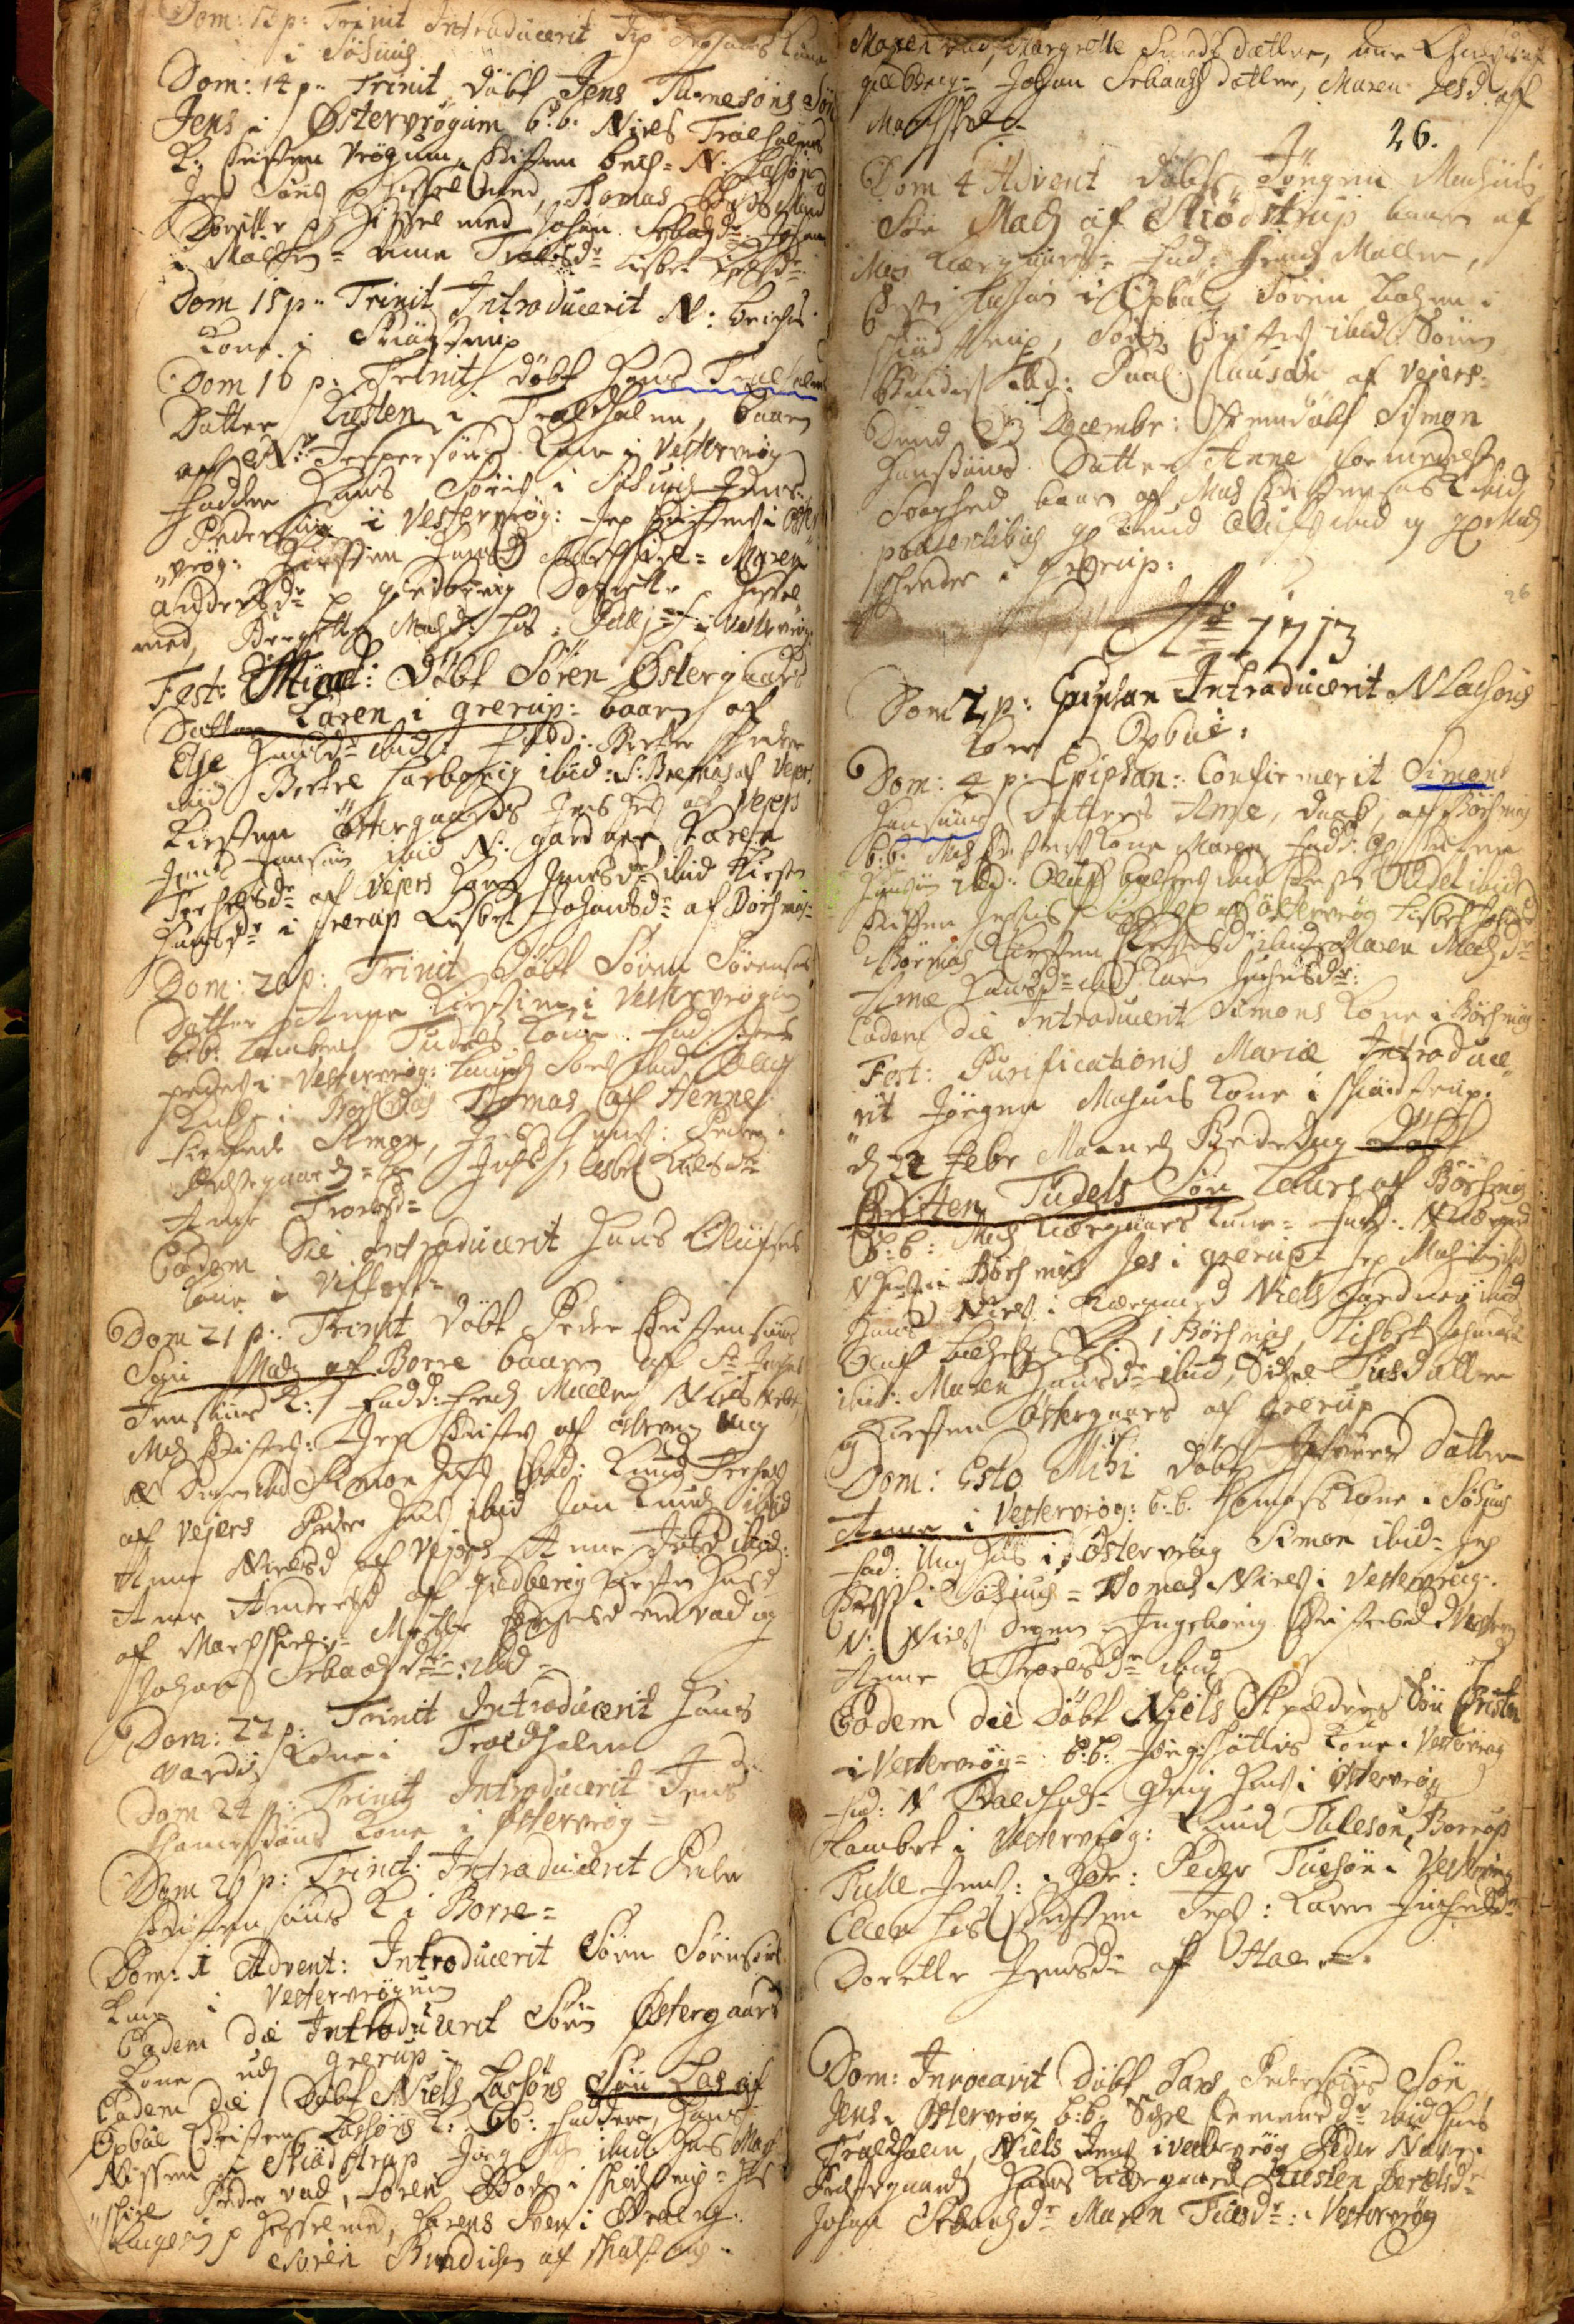Dom: 13 p: Trinit Introducerit Jep Jepsøns Kone
i Søhuus.
Dom: 14 p: Trinit døbt Jens Thomesøns Søn
Jens i Østervrøgum b.b. Niels Troldholms
K: Christen Vrøgum, Christen Bech: N: Lassøn
Jep Sørens p Hisselmed, Thomas Bodz Mand
Dorette p Hissel med, Johan Sebadzdr. Johanne
i Møllen: Anne Trolsdr Lisbet Kielsdr
Dom 15 p.. Trinit Introducerit N: Brichs
Kone i Skiødstrup
Dom 16 p: Trinit. døbt Hans Trolholms
Datter Kiesten i Troldholm, baaren
af N: Jespersøns Kone i Vestervrøg
Fadder Hans Sørens i Søhuus, Jens
Pedersøn i Vestervrøg: Jep Christensøn i Øster¬
vrøg: Kirsten Hansdr Marckschiel: Maren
Andersdr p Giedberig Dorette p Hissel¬
med, Bergitte Madzdr hos Pallj ## Vestervrøg:
Fest: Micael: Døbt Søren Østergaards
Datter Karen i Grerup: baaren af
Else Hansdr ibid: Fadd: Bertel Schreder
ibid Bertel Harborig ibid: S: Bremos af Vejers
Kirsten Østergaards Jes ## af Vejers
Jens Jensøn ibid N: Garders Karen
Terchelsdr af Vejers Karen Jensdr ibid Kirsten
Hansdr i Grerup, Lisbet Johansdr af Børsmøs:
Dom: 20 p: Trinit døbt Søren Sørensens
Datter Anne Kirstine i Vestervrøgum
b:b: Lambert Tudels Kone. Fad: Jes
pedersen i Vestervrøg: Lauridz ##s bid, Oluf
Knuds i Børsmos Thomas af Henne
tienende Simon, Jens Guns: Peder i
Præstegaard= ## ## Lisbet: Kielsdr
Anne Troelsd=
Eodem Die Introducerit Hans Olufsens
Kone i Viftoft=
Dom21 p: Trinit døbt Peder Christensøns
Søn Madz af Borre baaren af Sr Jacob
Tenskiers K: Fadd: Fred## M##, Niels Niels,
M##s Christens:  Jep Christens af Østervrog ##
N Degn ibd Simon Hans ibid: Knud Terchels
af Vejers Peder Hans ibid J## Knuds ibid
Anne Nielsd af Vejers, Anne J##sd ibid:
Anne Andersd af Giedberig Kirsten Hansd
af Marchschiel: = Mette E##sd ved Vad og
Johan Sebædzr: ibd=
Dom: 22 p: Trinit Introducerit Hans
Vardis Kone i Troldholm
Dom 24 p: Trinit Introducerit Jens
Thomessøns Kone i Østervrøg=
Dom.26 p.Trinit. Introducerit Peder
Christensøns K i Borre=
Dom:1 Advent: Introducerit Søren Sørensøns
Kone i Vestervrøgum
Eodem Die Introducerit Søren Østergaards
Kone udi Grerup.
Eodem Die Døbt Niels Lassøns Søn Las af
Oxbøl Christen Lassøns K: bb: Faddere, Hans
Nissen i Skiødstrup, Jørgen ## ibid, Hans March
schiel Peder vad, Søren Bods i Scheilstrup= Jes##
Lauges p Hesselmed, Lorens Sven## i Broeng,
Søren Bendiches af Skeelstrup
26
26
Maren ##, Margrette Svendsdatter, Ane Anders## af
Gielbereg= Johan Sebaass Datter, Maren Jesd af
Marchschiel=
Dom 4 Advent døbt Jørgen Madzøns
Søn Madz af Skiødstrup baaren af
Maz## Kiærgaards= Fad: Frandz Møller,
Christen Lassøn i Oxbøl, Søren Bachen i
Schiødstrup, Søren Christensen ibid: Søren
Bundes ibd, Poul Claus af Vejers:
Dend 23 Decembr: hiemdøbt Simon
Hansøns Datter Anne formedelst
Svaghed baaren af Madz Christensens K ibid
p## gl Knud Olufs ibid og gl Hr## Madz
Schreder i Grerup.
Ao 1713
Dom 2 p: Epiphan. Introducerit N Lassøns
Kone i Oxbøl:
Dom: 4 p: Epiphan: Confirmerit Simon
Hansøns Datters Anne, Daab af Børsmos
b:b. Madz Christens Kone Maren, Fad: gl Christen
Hansøn ibid: Oluf Bachens## ibid, Christen Tudel ibid
Christen Jepsses Søren Jep## af Østervrøg Lisbet Johansd
i Børsmos, Kirsten Christensdr## ibid, Maren Madzdr
Anne Lausdr ibid, Karen ##chesdr:
Eodem Die Introducerit Simons Kone i Børsmos.
Fest. Purificationis Mariæ Introduce¬
rit Jørgen Madsens Kone i Schiødstrup
D 22 Febr Maaneds Bededag døbt
Christen Tudels Søn Laurs af Børsmos
b: b: Madz Kiærgaards Kone. Fad: N. Kiærgaard
N. Hansen i Børsmos, Jes i Grerup Jep Ma## ibid
Hans Niels i Kiærgaard, Niels ## ibid
Oluf Belh## ## i Børsmos, Lisbet Johansd
ibid: Maren Hansdr ibid, Sidtzel Tusdatter,
Kirsten Østergaards af Grerup
Dom. Esto Mihi døbt Ifvers Datter
Anne i Vestervrøg: b.b. Thomas Kone i Søhuus
Fad: Ung H## i Østervrøg Simon ibid= Jep
Bac## i Søhuus= Thomas Nielsen i Vestervrøg:
N: Niels Degen Ingeborig Christensd Vestervrøg##
Anne Troelsdr ibid.
Eodem Die Døbt Niels Skredders Søn Christen
i Vestervrøg= b:b: Jørgen Schøttis Kone i Vestervrøg
Fad: N Troldho= Gunnj Hans i Østervrøg
Lambert i Vestervrøg: Knud Tulesøn Borrup,
Tulle Jensen i Hoe, Peder Tuesøn i Vestervrøg.
Ellen hos Christen Jeps: Karen J##chesdr
Dorette Jensdr af Hoe=.
Dom. Invocavit Døbt Hans Pedersøns Søn
Jens i Østervrøg. b:b: Sidsel Clemensdr ibid Hans
Troldholm, Niels Jens i Vestervrøg Peder Nielsen 
Præstegaard, Hans Kiærgaard, Kiesten Bertelsdr
Johan Sebæsdr Maren Tuesdr: Vestervrøg
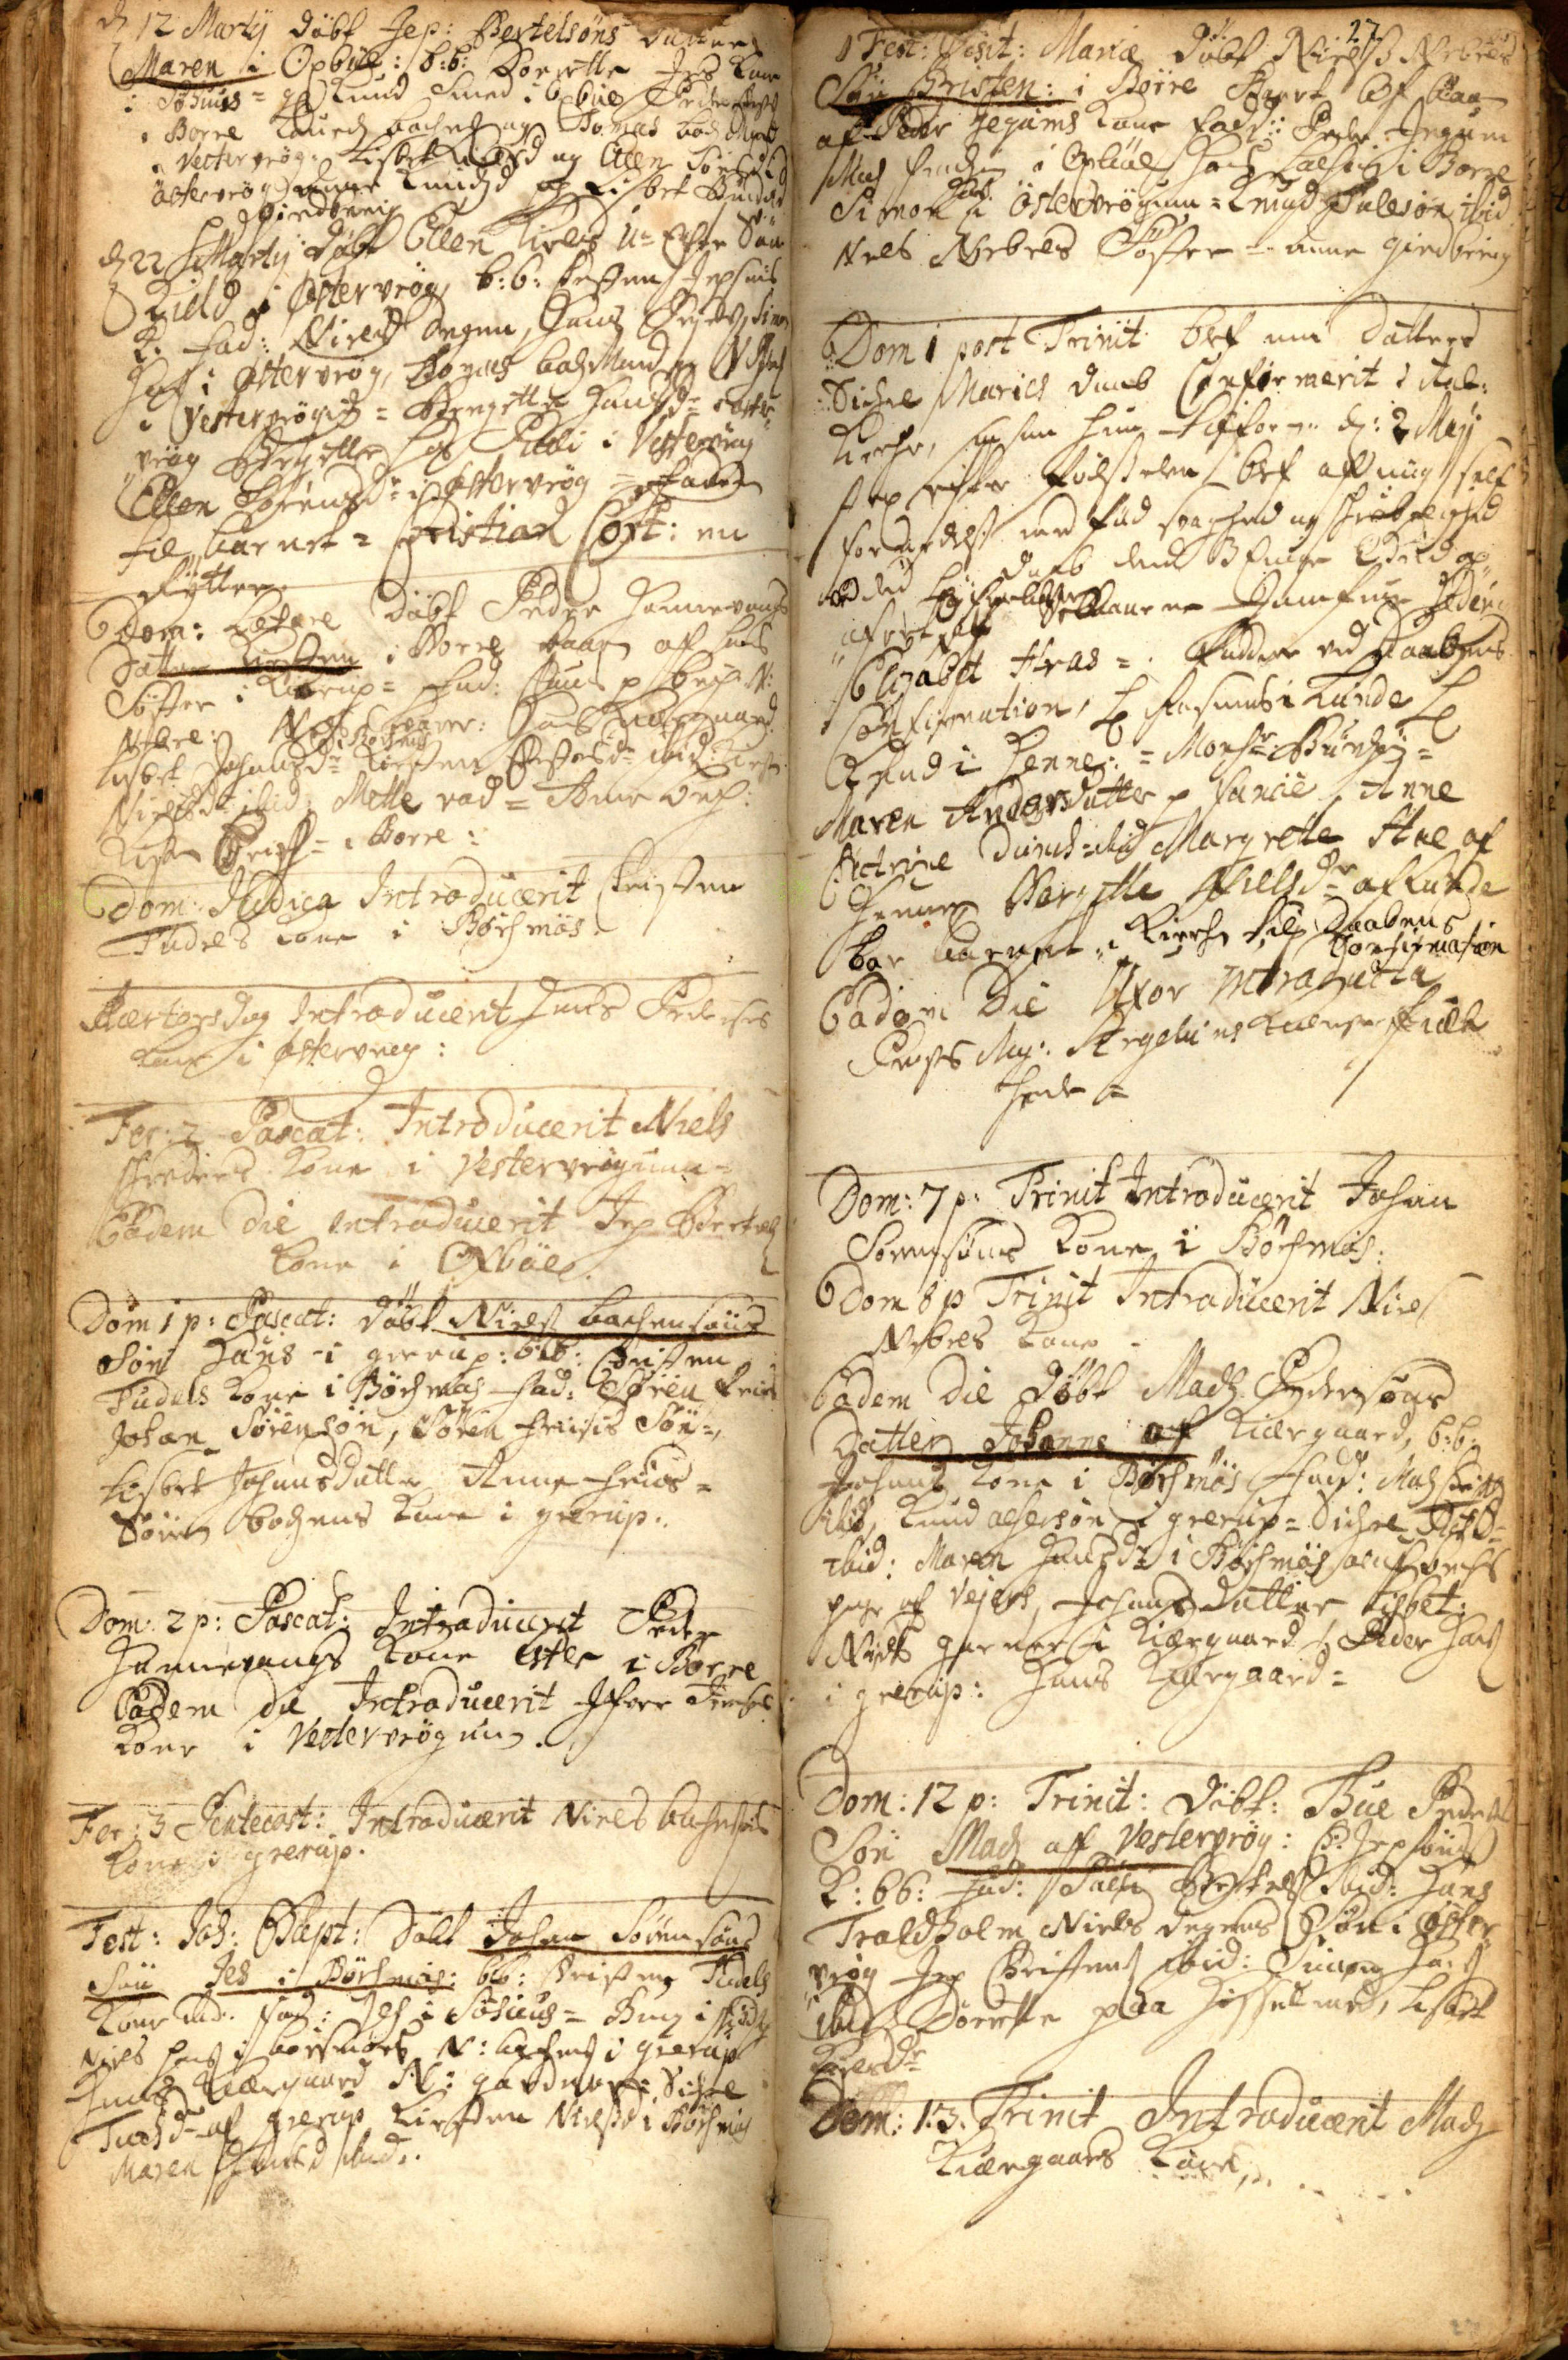d 12 Martij døbt Jep: Bertelsøns Datter
Maren i Oxbøl: b:b: Dorrette Jes Kone
i Søhuus= gl## Knud Smed i Oxbøl, Peder ##
i Borre Lauridz Baches og Thomas BochMand##
i Vestervrøg:  Lisbet Kielsd og Ellen Sørensd## i
Østervrøg Anne Knudsd og Lisbet Bundesd
Giedberig
d 22 Martij Døbt Ellen Kiels u=ægte Søn
Kield i Østervrøg, b:b: Christen Jepsøns
K. Fad: Niels Degen, Hans ## Simon
Hans i Østervrøg, Thomas BodzMand og N Jes##
i Vestervrøg= Bergette Hansdr ##ter¬
vrøg Bergette hos Palli i Vestervrøg
Ellen Sørensdr i Østervrøg= Fader
til Barnet= Christian Cort: en
Rytter.
Dom: Lætare Døbt Peder Hannevangs
Datter Kirsten i Borre baaren af hans
Søster i Kierup= Fad: Claus p Brich N:
Nebel: Niels S##: Hans Kiærgaard,
i Bochm##
Lisbet Johansdr Kirsten Christensdr ibid: Kirsten
Nielsdr ibid: Mette vad= Anne Bech:
Kirsten Christ##= i Borre:
Dom: Judica Introducerit Christen
Tudels Kone i Børsmøs.
Skærtorsdag Introducerit Hans Pedersens
Kone i Østervreg:
Fert: 2 Pascat: Introducerit Niels
Schreders Kone i Vestervrøgum=
Eodem Die Introducerit Jep Bertels
Kone i Oxbøl.
Dom1 p: Pascat: døbt Niels Bachensøns
Søn Hans i Grerup: b:b: Christen
Tudels Kone i Børsmos Fad: Søren Bris##
Johan Sørensøn, Søren Friisis Søn=,
Lisbet Johansdatter, Anne Friis¬
Søren Bodsens Kone i Grerup.
Dom: 2 p: Pascat: Introducerit Peder
Hannevangs Kone Ester i Borre
Eodem Die Introducerit Ifver Jensens
Kone i Vestervrøgum.
Fer: 3 Pentecost: Introducerit Niels Bachens
Kone i Grerup.
Fest: Joh: Bapt: Døbt Johan Sørensøns
Søn Jes i Børsmøs: b:b: Christen Tudels
Kone ibid: Fad: Jes i Søhuus= Bunj i Schiødst##
Niels Hans i Børsmoes, N. Bachens i Grerup,
Hans Kiærgaard, N. Garder ##, Sisel
Tuchdr af Grerup, Kirsten Nielsd i Børsmøs,
Maren Hansd ibid.
27
27
Fest.Visit.Mariæ døbt Niels Nebels
Søn Christen: i Borre, Baaret ##f ##
af Peder Jegums Kone Fadd: Peder Jegum
Mads Frands i Oxbøl Hans ##al i Borre
Simon Hans i Østervrøgum= Knud Tulesøn ibid
Niels Nebels Søster ## Anne Giedberig
Dom 1 post Trinit blef min Datter
Sidsel Maries Daab Confirmerit i Aal:
Kirche, saasom hun tilforn" d: .2 Majj
strax efter Fødselen blef af mig self
formedelst medfød Svaghed og Schrøbelighed
ved## den## H: Daab ## 3 ## op¬
## F##
ført af Velbaarne Jomfrue Hedevig
Elisabet Hvas=. Faddere ved Daabens
Confirmation, Hl## Rasmus i Lunde, Hl##
Knud i Henne:= Monsr Bundes=
Maren Andersdatter p Fanøe, Anne
Chatrine## D##= ibid, Margrette Hoe af
Henne Bergitte Nielsdr af Lunde
bar Barnet i Kirchen til Daabens
Confirmation
Eodem Die Uxor introduce##
## ##: ## ## ##
H##
Dom: 7 p: Trinit Introducerit Johan
Sørensøns Kone i Børsmøs
Dom 8 p Trinit Introducerit Niels
Nebels Kone.
Eodem Die døbt Madtz Pedersøns
Datter Johanne af Kiærgaard, b:b:
Johans Kone i Børsmøs Fadd: Madz Christens##
ibid, Knud Achersøn i Grerup= Sidsel ##
ibid: Maren Hansdr i Børsmøs ##
## af Vejers, Johans Datter Lisbet:
Niels Garner## i Kiærgaard, Peder Hans
i Grerup: Hans Kiærgaard=
Dom: 12 p: Trinit: Døbt Thue Peders
Søn Madz af Vestervrøg: Ch: Jepsøns
K: bb: Fad: Palli Bertelsen ibid Hans
Troldholm, Niels Degens Søn i Øster¬
vrøg Jep Christens ibid: Simon Hans
ibid, Dørrette paa Hisselmed, Lisbet
Kielsdr
Dom: 1.3. Trinit Introducerit Madz
Kiærgaards Kone,